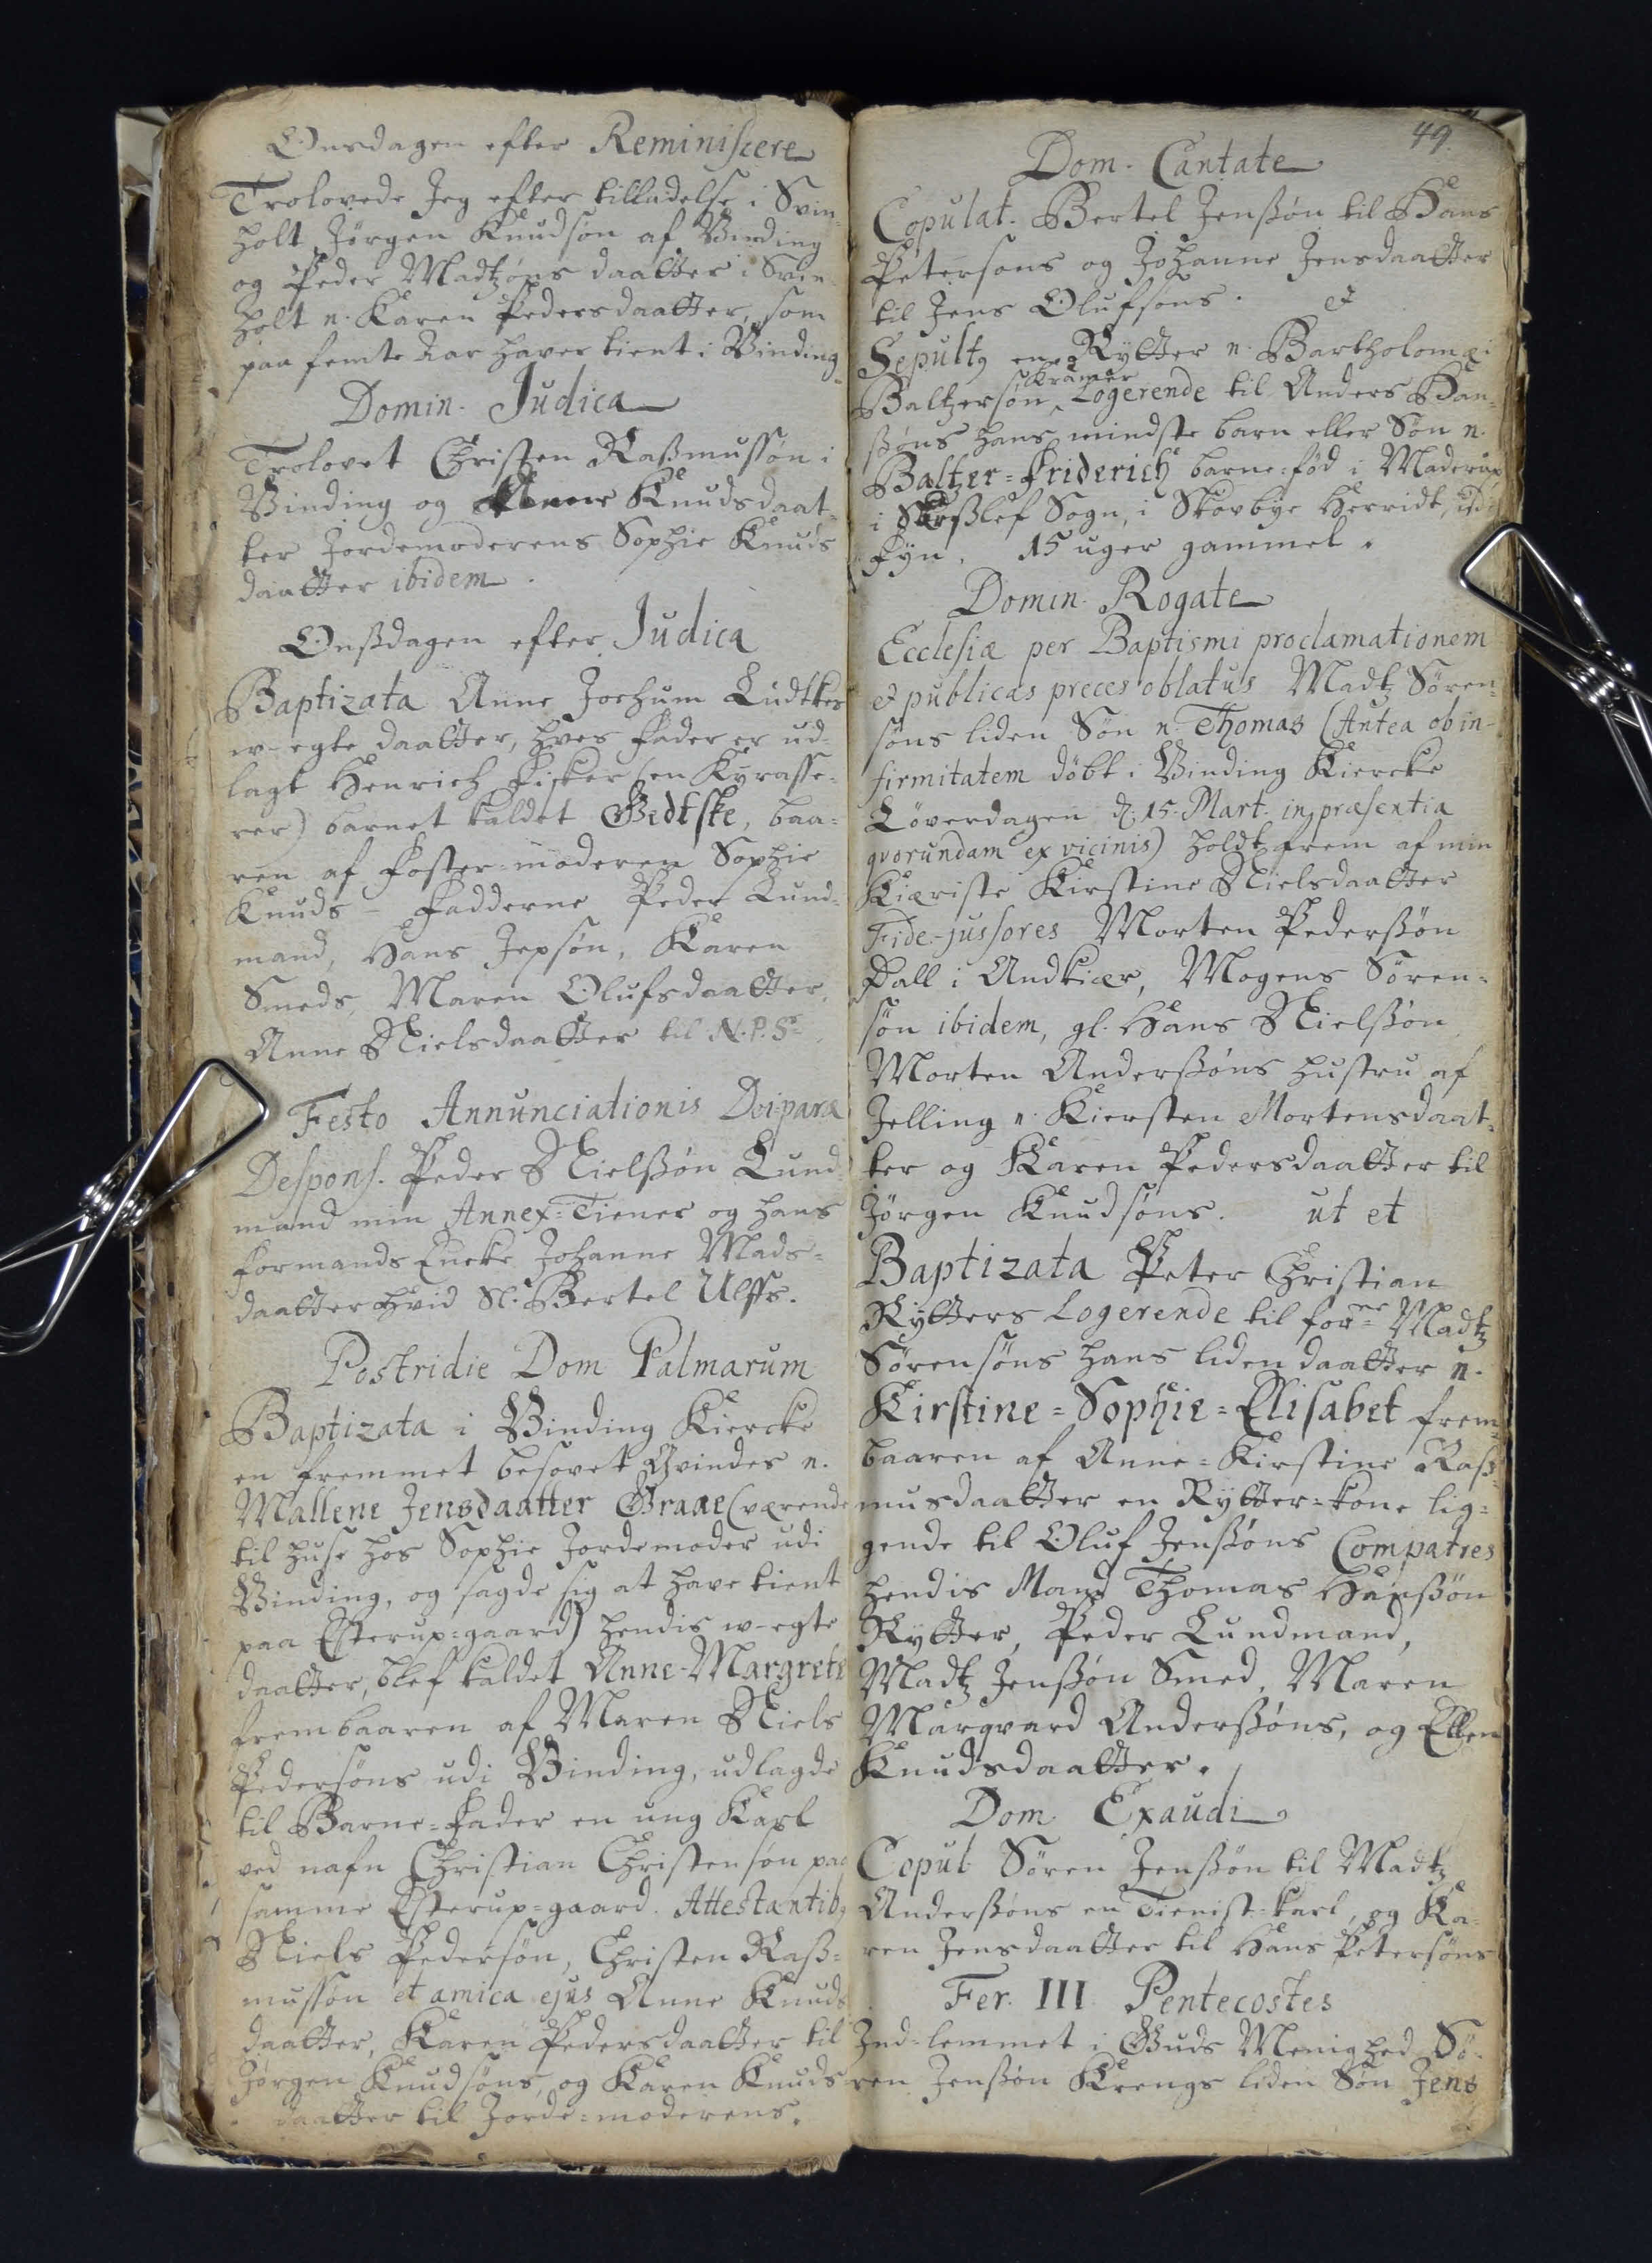Onsdagen efter Reminiscere
Trolovede Jeg efter tilladelse i Svin¬
holt Jørgen Knudsøn af Vinding
og Peder Madtzøns daatter i Svin
holt n. Karen Pedersdaatter, som
paa femte Aar haver tient i Vinding
Dom. Judica
Trolovet Christen Rasmussøn i
Vinding og Anne## Knudsdaat¬
ter Jordemoderens Sophie Knuds
daatter ibidem
Onsdagen efter Judica
Baptizata Anne Jochum Ludtkes 
w-egte daatter, hves Fader er ud¬
lagt Henrich Fisker (en Kyrasse¬
rer) barnet kaldet Gedtske, baa¬
ren af Foster=moderen Sophie
Knuds - Faddere Peder Lund¬
mand, Hans Jepsøn, Karen
Smeds. Maren Olufsdaatter.
Anne Nielsdaatter til N.P.Ss
Festo Annunciationis Deiparæ
Despons. Peder Nielsøn Lund¬
mand min Annex=Tiener og hans 
Formands Encke Johanne Mads¬
daatter Hvid Sl. Bertel Ulffs.
Postridie Dom Palmarum
Baptizata i Vinding Kiercke
en fremmet besovet Qvindes n.
Mallene Jensdaatter Graae (værende
til huse hos Sophie Jordemoder udi
Vinding, og sagde sig at have tient
paa Esterup=gaard) hendis w-egte
daatter, blef kaldet Anne-Margrethe
frembaaren af Maren Niels 
Pedersøns udi Vinding, udlagde
til Barne=fader en ung Karl
ved nafn Christen Christensen paa
samme Esterup=gaard. Attestantibq
Niels Pedersøn. Christen Rass¬
mussøn et amica ejus Anne Knuds
daatter, Karen Pedersdaatter til
Jørgen Knudsøns, og Karen Knuds
daatter til Jorde=moderens.
49.
Dom. Cantate
Copulat. Bertel Jenssøn til Hans
Petersons og Johanne Jensdaatter
til Jens Olufsøns.
Sepulta en Rytter n. Bartholomæ i
Kramer
Baltzersøn, Logerende til Anders Han¬
ssøns hans mindste barn eller Søn n.
Baltzer=Friderich, barne=fød i Maderup
i S##rsslef Sogn, i Skovbye Herridt, udi
Fyn. 15 uger gammel.
Dom. Rogate
Ecclesiæ per Baptismi proclamationem
et publicas preces oblatus Madtz Søren¬
søns liden Søn n. Thomas (Antea obin¬
firmitatem døbt i Vinding Kiercke 
Løverdagen d. 15. Mart. in præsentia
qvorundam ex vicinis) holdt frem af min
Kiæriste Kirstine Nielsdaatter
Fide-jussores Morten Pederssøn
Dall i Andkiær, Mogens Søren¬
søn ibidem, gl. Hans Nielssøn
Morten Anderssøns hustru af
Jelling n. Kiersten Mortensdaat¬
ter og Karen Pedersdaatter til
Jørgen Knudsøns. ut et.
Baptizata Peter Christian
Rytters, Logerende til forne Madtz
Sørensøns, hans liden daatter n.
Kirstine = Sophie = Elisabet, frem
baaren af Anne = Kirstine Ras¬
musdaatter en Rytter=Kone lig¬
gende til Oluf Jenssøns Compatres
hendis Mand Thomas Hanssøn
Rytter, Peder Lundmand,
Madtz Jenssøn Smed, Maren
Marqvard Anderssøns, og Ellen
Knudsdaatter.
Dom. Exaudi
Copul. Søren Jenssøn til Madtz
Anderssøns en Tieneste=karl, og Ka¬
ren Jensdaatter til Hans Petersøns
Fer. III Pentecostes
Ind=lemmet i Guds Menighed Sø¬
ren Jenssøn Krengs liden Søn Jens
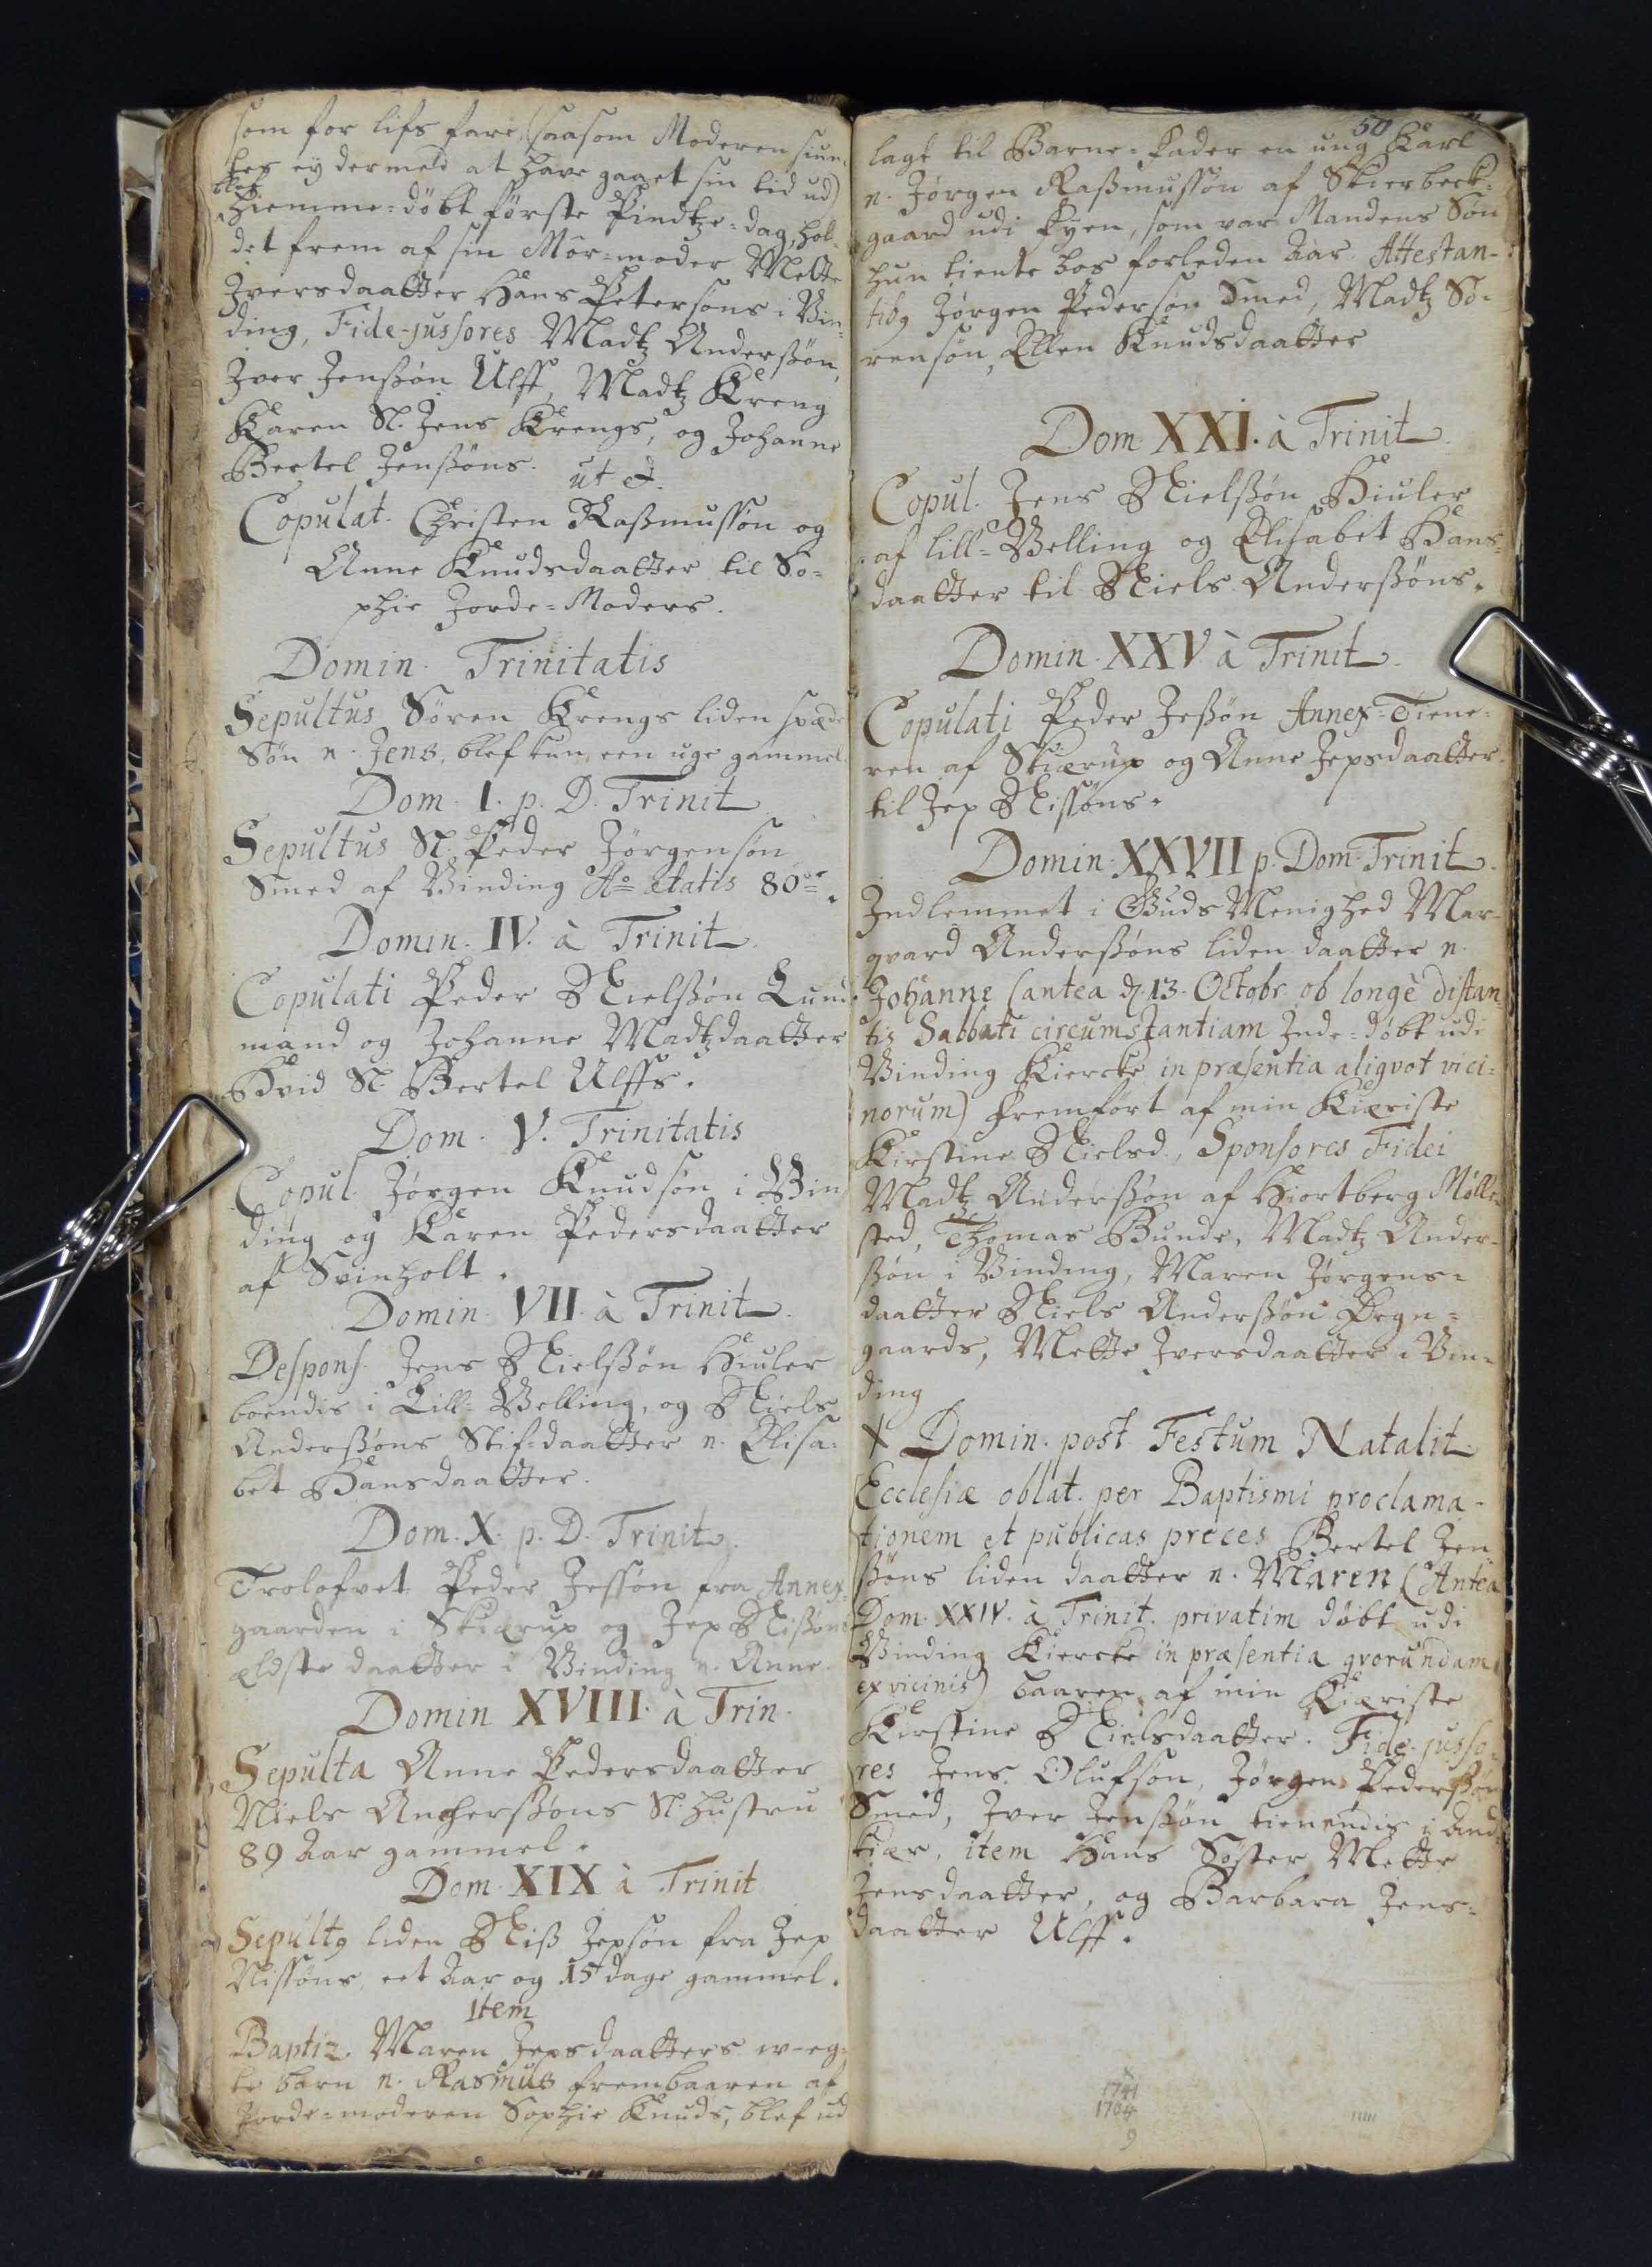som for lifs fare (saasom Moderen siun
tes ey dermed at have gaaet sin tid ud) 
blef
hiemme=døbt første Pindtze=dag, hol¬
det det frem af sin Mor=moder Mette
Iversdaatter Hans Petersøns i Vin¬
ding, Fide-jussores Madtz Anderssøn
Iver Jenssøn Ulff, Madtz Kreng
Karen Sl. Jens Krengs, og Johanne
Bertel Jenssøns. ut et.
Copulat. Christen Rasmussøn og
Anne Knudsdaatter til So¬
phie Jorde=moders
Domin. Trinitatis
Sepultus Søren Krengs liden spæde
Søn n. Jens, blef kun een uge gammel
Dom. 1. p. D. Trinit
Sepultus Sl. Peder Jørgensøn 
Smed af Vinding Ao ætatis 80
Domin. IV. a Trinit
Copulati Peder Nielssøn Lund
mand og Johanne Madtzdaatter 
Hvid Sl. Bertel Ulffs.
Dom. V. Trinitatis
Copul. Jørgen Knudsøn i Vin
ding og Karen Pedersdaatter
af Svinholt
Domin. VII. a Trinit
Despons. Jens Nielssøn Hiuler
boendis i Lill= Velling, og Niels
Anderssøns Stif=daatter n. Elisa¬
bet Hansdaatter.
Dom. X. p. D. Trinit
Trolofvet Peder Jessøn fra Annex
gaarden i Skiærup og Jep Nissøns
ældste daatter i Vinding n. Anne.
Domin XVIII. a Trinit
Sepulta Anne Pedersdaatter 
Niels Ancherssøns Sl. hustru
89 Aar gammel.
Dom. XIX a Trinit.
Sepultq liden Niss Jepsøn fra Jep
Nissøns, eet Aar og 15 dage gammel.
item
Baptiz. Maren Jepsdaatters w-eg¬
te barn n. Rasmus frembaaren af
Jorde=moderen Sophie Knuds, blef ud
50
lagt til Barne = Fader en ung Karl
n. Jørgen Rassmussøn af Skierbeck¬
gaard udi Fyen, som var Mandens Søn
hun tiente hos forleden Aar Attestan¬
tibq Jørgen Pedersøn Smed, Madtz Sø¬
rensøn, Ellen Knudsdaatter
Dom. XXI. a Trinit
Copul. Jens Nielssøn Hiuler
af Lill= Velling og Elisabet Hans¬
daatter til Niels Anderssøns.
Domin XXV a Trinit
Copulati Peder Jessøn Annex=Tiene¬
ren af Skiærup og Anne Jepsdaatter
til Jep Nissøns.
Domin XXVII p. Dom. Trinit.
Indlemmet i Guds Menighed Mar¬
qvard Anderssøns liden daatter n.
Johanne (antea d. 13 Octobr ob longe distan¬
tis Sabbati circumstantiam Inde = døbt udi
Vinding Kiercke in præsentia aligvot vici¬
norum) fremført af min Kiæriste 
Kirstine Nielsd, Sponsores Fidei
Madtz Anderssøn af Hiortberg Mølle¬
sted, Thomas Bunde, Madtz Ander¬
ssøn i Vinding, Maren Jørgens¬
daatter Niels Anderssøn Degn¬
gaards, Mette Iversdaater i Vin¬
ding
Domin. post Festum Natalit
Ecclisiæ oblat. per Baptismi proclama¬
tionem et publicas preces Bertel Jen¬
ssøns liden daatter n. Maren (Antea
Dom. XXIV a Trinit. privatim døbt udi
Vinding Kiercke in præsentia qvorundam 
ex vicinis) baaren af min Kiæriste
Kirstine Nielsdaatter. Fide-jusso¬
res Jens Olufsøn, Jørgen Pederssøn
Smed, Iver Jenssøn tienendis i And
kiær, item hans Søster Mette
Jensdaatter, og Barbara Jens¬
daatter Ulff.